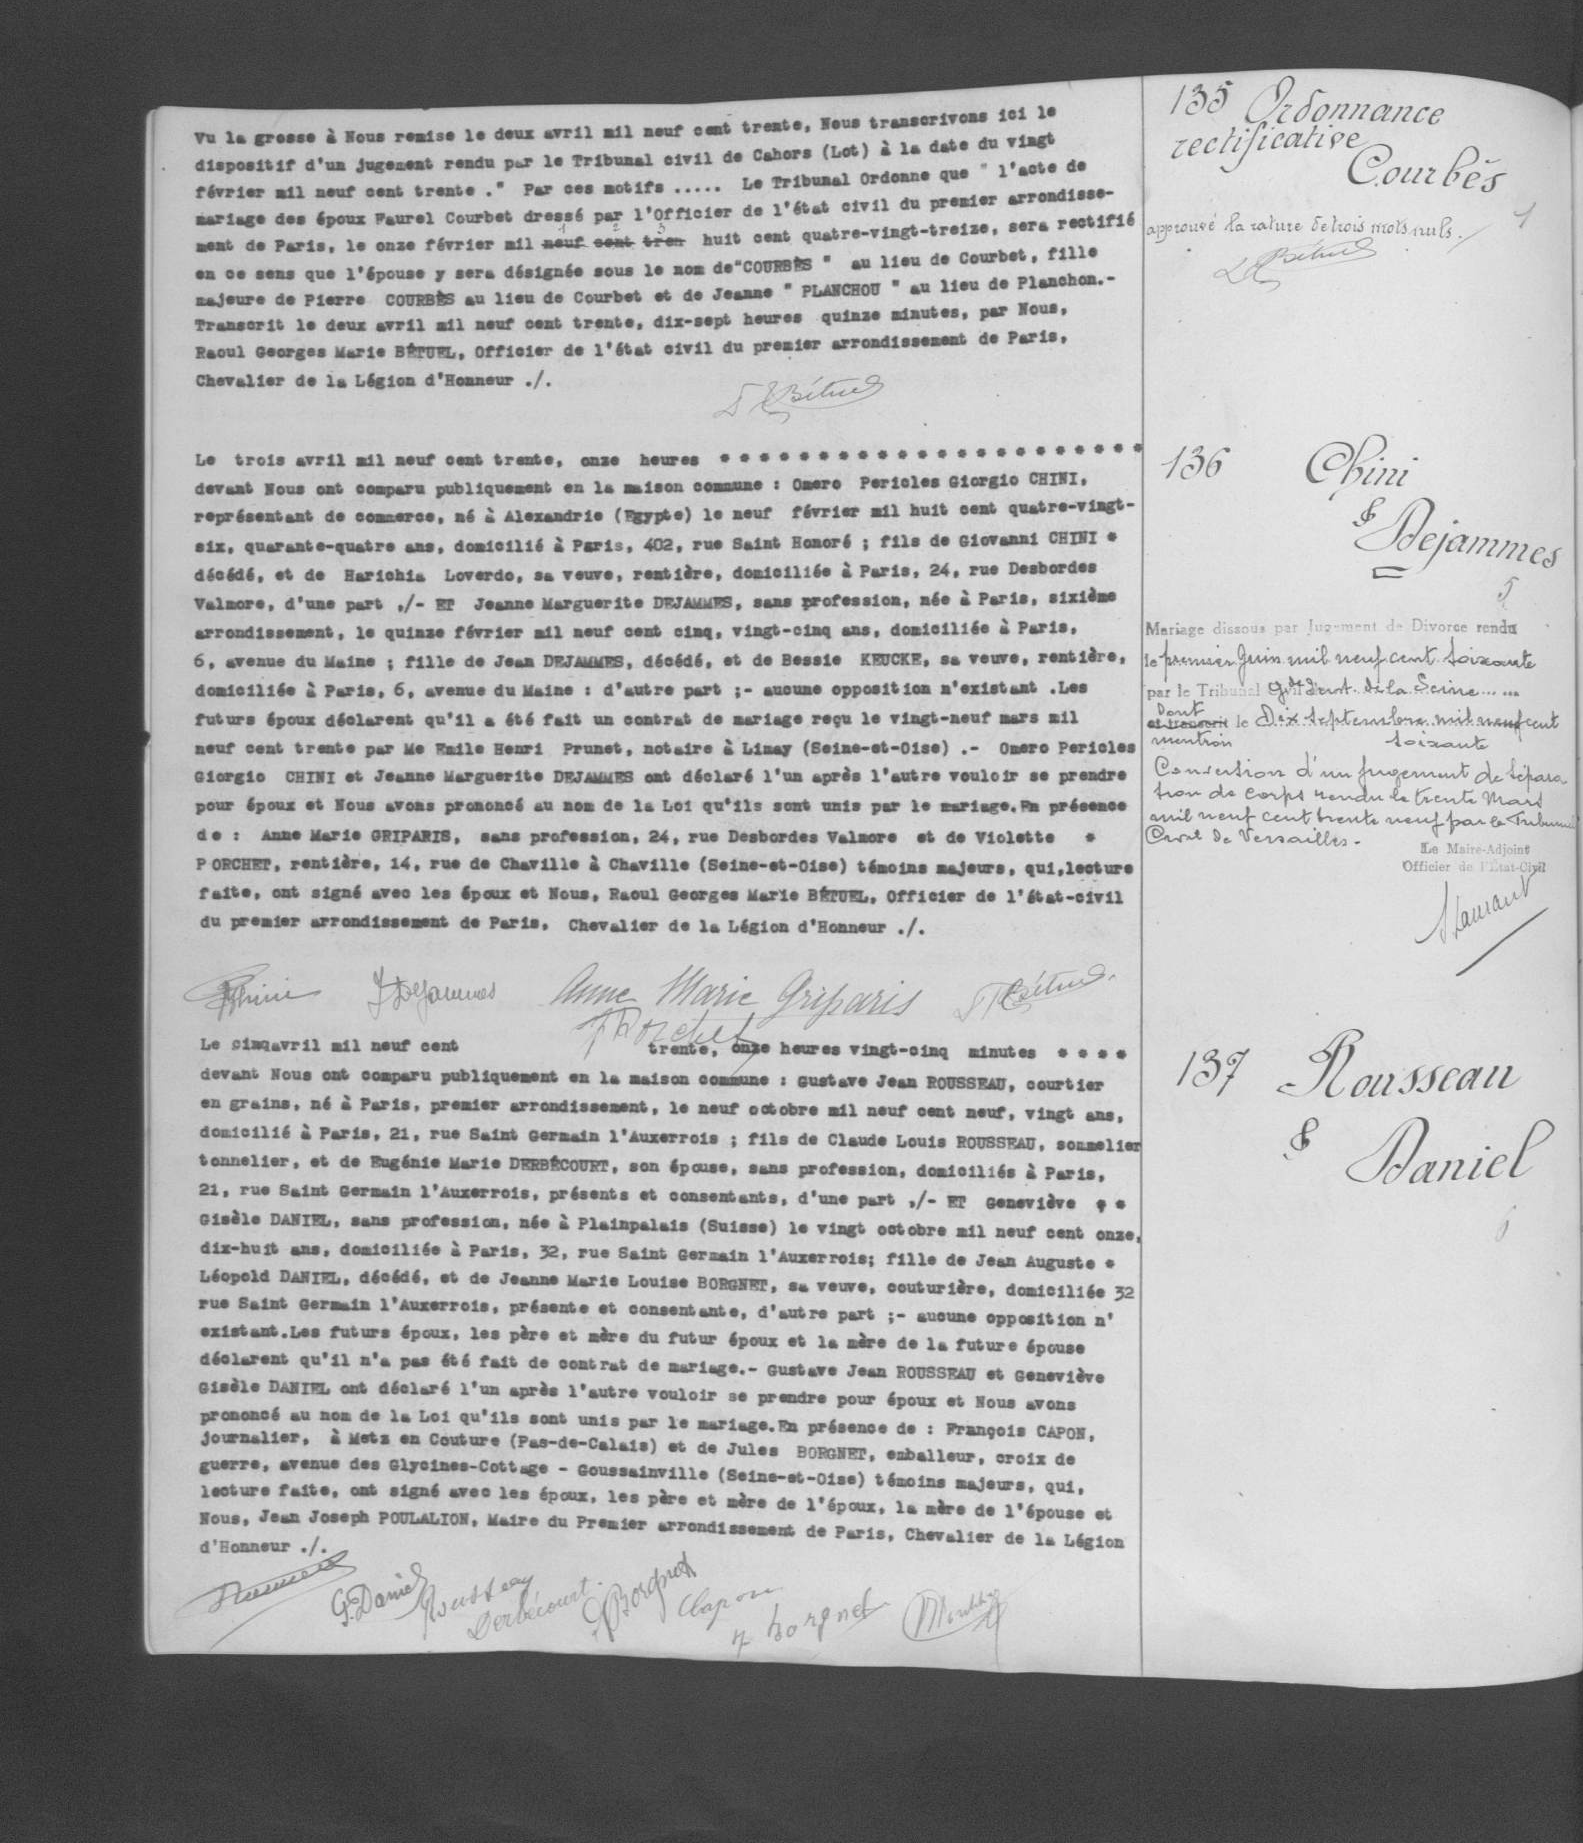Vu la grosse à Nous remise le deux avril mil neuf cent trente, Nous transcrivons ici le
dispositif d'un jugement rendu par le Tribunal civil de Cahor (Lot) à la date du vingt
février mil neuf cent trente." Par ces motifs..... Le Tribunal Ordonne que l'acte de
mariage des époux Faurel Courbet dressé par l'Officier de l'état civil du premier arrondisse-
ment de Paris, le onze février mil neuf cent tren huit cent quatre-vingt-treize, sera rectifié
en ce sens que l'épouse y sera désignée sous le nom de" COURBES" au lieu de Courbet, fille
majeure de Pierre COURBES au lieu de Courbet et de Jeanne" PLANCHOU" au lieu de Planchon .-
Transcrit le deux avril mil neuf cent trente, dix-sept heures quinze minutes, par Nous,
Raoul Georges Marie BETUEL, Officier de l'état civil du premier arrondissement de Paris,
Chevalier de la Légion d'Honneur ./.
Le trois avril mil neuf cent trente, onze heures ****
devant Nous ont comparu publiquement en la maison commune: Omero Pericles Giorgio CHINI,
représentant de commerce, né à Alexandrie (Egypte) le neuf février mil huit cent quatre vingt
six, quarante-quatre ans, domicilié à Paris, 402, rue Saint Honoré; fils de Giovanni CHINI *
décédé, et de Harichia Loverdo, sa veuve, rentière, domiciliée à Paris, 24, rue Desbordes
Valmore, d'une part ,/-ET Jeanne Marguerite DEJAMMES, sans profession, née à Paris, sixième
arrondissement, le quinze février mil neuf cent cinq, vingt-cinq ans, domiciliée à Paris,
6, avenue du Maine; fille de Jean DEJAMMES, décédé, et de Besssie KEUCKE, sa veuve, rentière,
domiciliée à Paris, 6, avenue du Maine: d'autre part ;-aucune opposition n'existant .Les
futurs époux déclarent qu'il a été fait un contrat de mariage reçu le vingt-neuf mars mil
neuf cent trente par Me Emile Henri Prunet, notaire à Limay (Seine-et-Oise) .-Omero Pericles
Giorgio CHINI et Jeanne Marguerite DEJAMMES ont déclaré l'un après l'autre vouloir se prendre
pour époux et Nous avons prononcé au nom de la Loi qu'il sont unis par le mariage. En présence
de: Anne Marie GRIPARIS, sans profession, 24, rue Desbordes Valmore et de Violette *
PORCHET, rentière, 14, rue de Chaville à Chaville (Seine-et-Oise) témoins majeurs, qui, lecture
faite, ont signé avec les époux et Nous, Raoul Georges Marie BETUEL, Officier de l'état-civil
du premier arrondissement de Paris, Chevalier de la Légion d'Honneur ./.
Le cinq avril mil neuf cent trente, onze heures vingt-cinq minutes ****
devant Nous ont comparu publiquement en la maison commune: Gustave Jean ROUSSEAU, courtier
en grains, né à Paris, premier arrondissement, le neuf octobre mil neuf cent neuf, vingt ans,
domicilié à Paris, 21, rue Saint Germain l'Auxerrois; fils de Claude Louis ROUSSEAU, sommelier
tonnelier, et de Eugénie Marie DERBECOURT, son épouse, sans profession, domiciliés à Paris,
21, rue Saint Germain l'Auxerrois, présents et consentants, d'une part ,/-ET Geneviève * *
Gisèle DANIEL, sans profession, née à Plainpalais (Suisse) le vingt octobre mil neuf cent onze,
dix-huit ans, domiciliée à Paris, 32, rue Saint Germain l'Auxerrois; fille de Jean Auguste *
Léopold DANIEL, décédé, et de Jeanne Marie Louise BORGNET, sa veuve, couturière, domiciliée
rue Saint Germain l'Auxerrois, présente et consentante, d'autre part ;-aucune opposition n'
existant. Les futurs époux, les père et mère du futur époux et la mère de la future épouse
déclarent qu'il n'a pas été fait de contat de mariage .-Gustave Jean ROUSSEAU et Geneviève
Gisèle DANIEL ont déclaré l'un après l'autre vouloir se prendre pour époux et Nous avons
prononcé au nom de la Loi qu'ils sont unis par le mariage. En présence de: François CAPON,
journalier, à Metz en Couture (Pas-de-Calais) et de Jules BORGNET, emballeur, croix de
guerre, avenue des Glycines-Cottage-Goussainville (Seine-et-oise) témoins majeurs, qui,
lecture faite, ont signé avec les époux, les père et mère de l'époux, la mère de l'épouse et
Nous, Jean Joseph POULALION, Maire du Premier arrondissement de Paris, Chevalier de la Légion
d'Honneur ./.
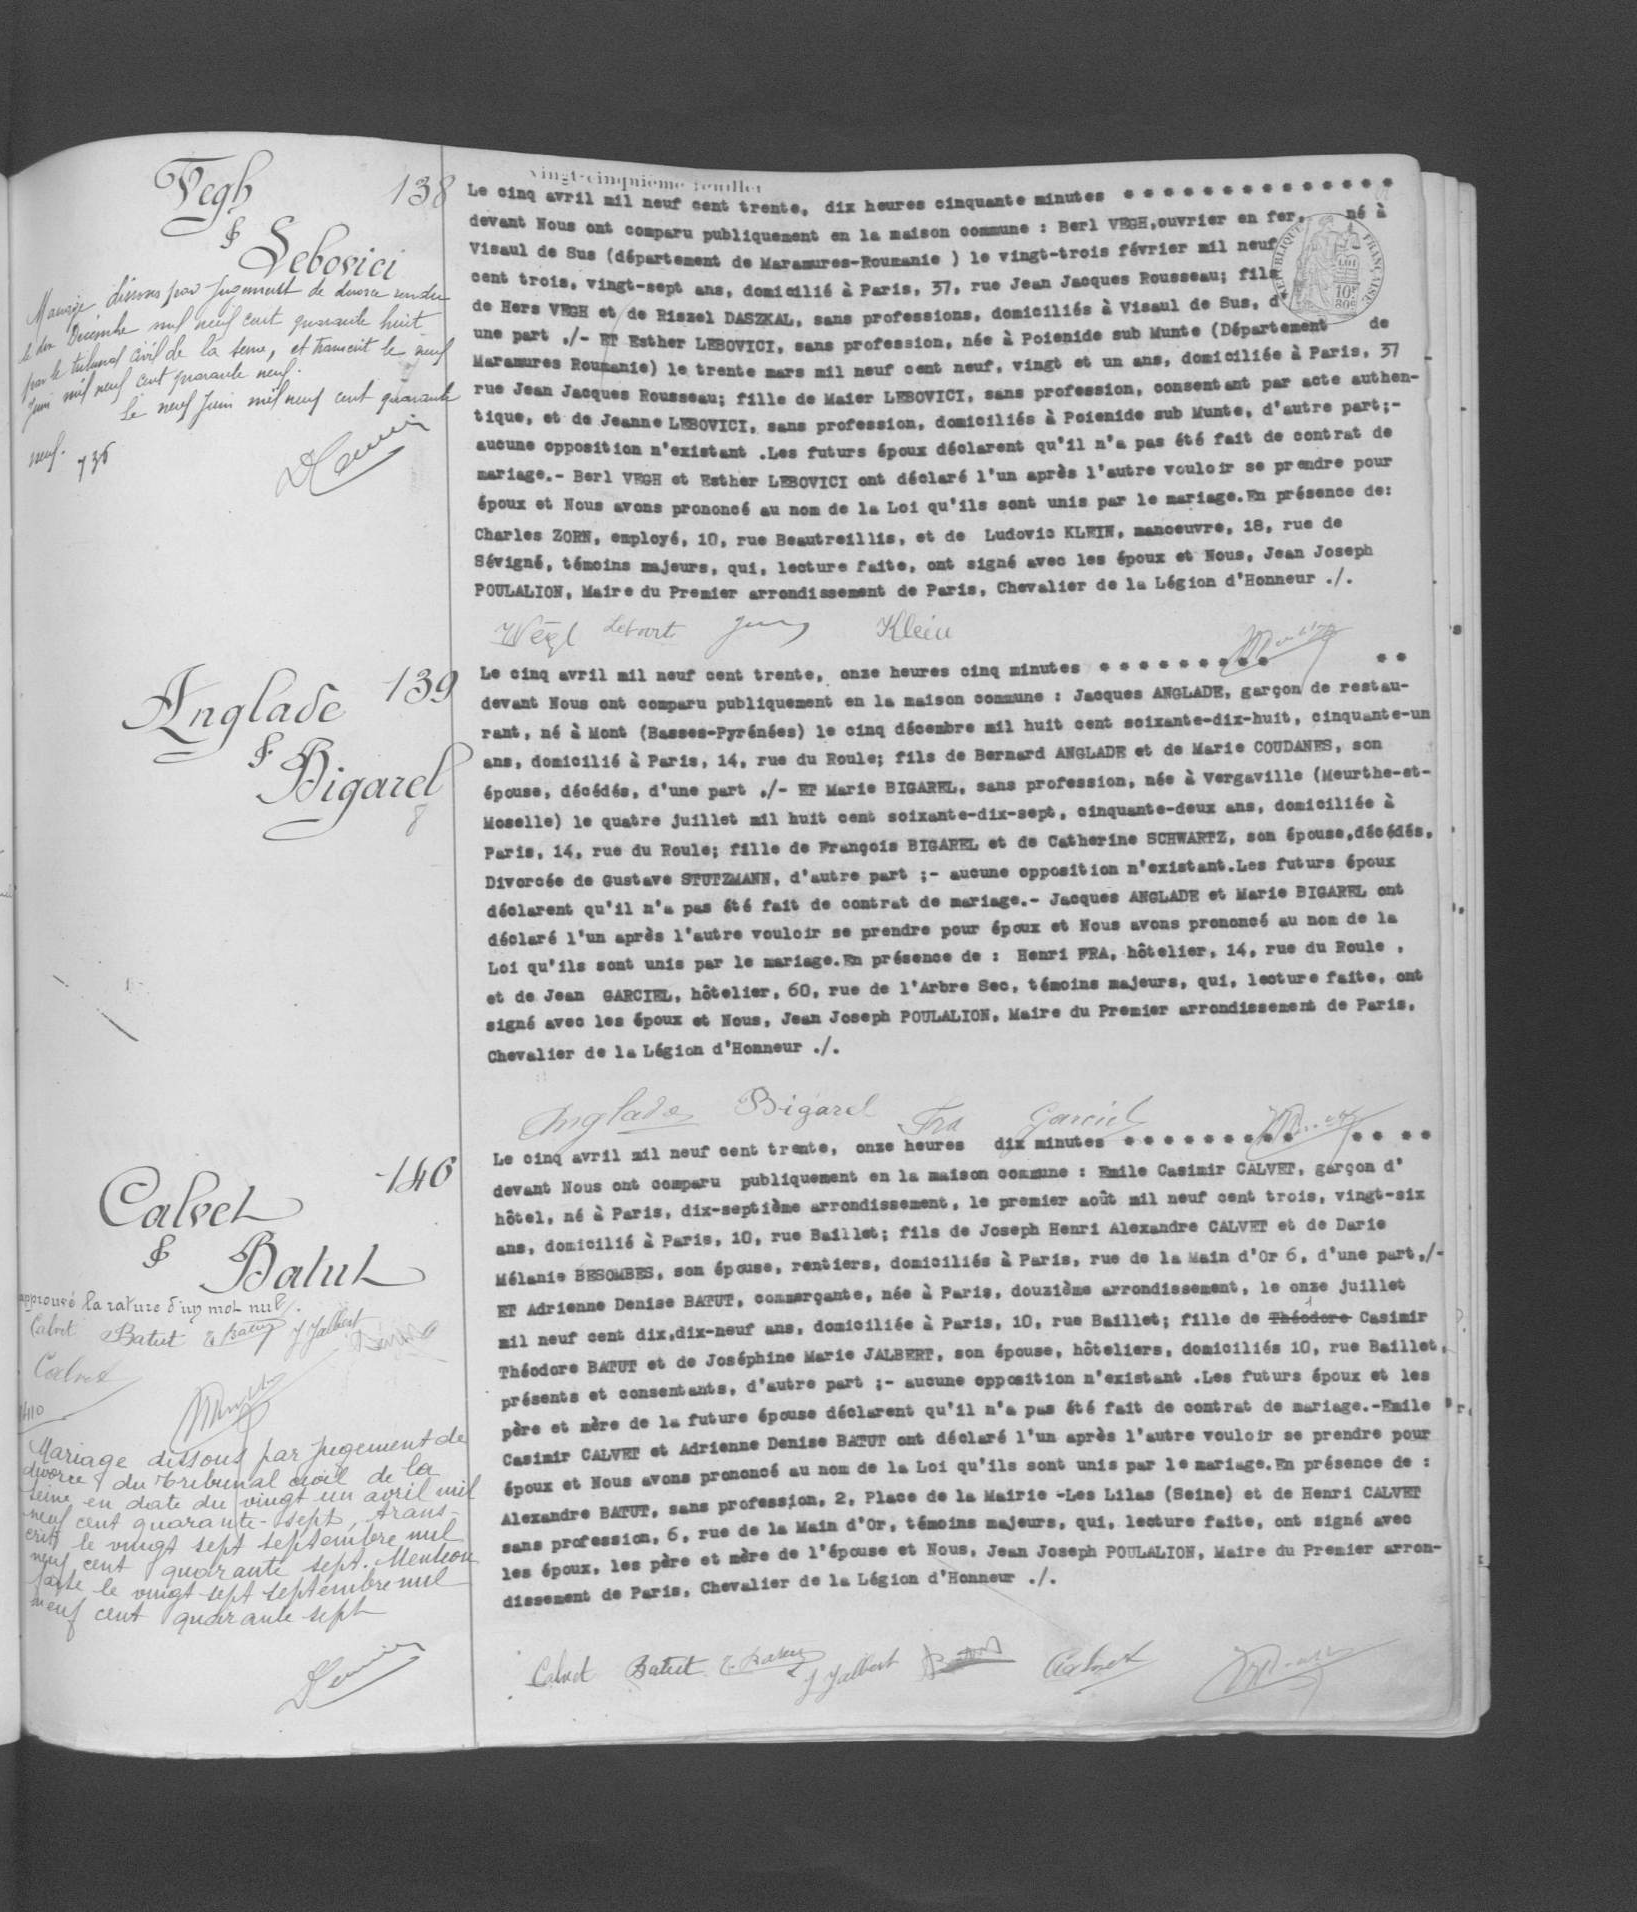Le cinq avril mil neuf cent trente, dix heures cinquante minutes ****
devant Nous ont comparu publiquement en la maison commune: Berl VEGH, ouvrier en fer, né à
Visaul de Sus (département de Maramures-Roumanie) le vingt-trois février mil neuf
cent trois, vingt-sept ans, domicilié à Paris, 37, rue Jean Jacques Rousseau; fils
de Hers VEGH et de Riszel DASZKAL, sans professions, domiciliés à Visaul de Sus, d'
une part ,/-ET Esther LEBOVICI, sans profession, née à Poienide sub Munte (Département de
Maramues Roumanie) le trente mars mil neuf cent neuf, vingt et un ans, domiciliée à Paris, 37
rue Jean Jacques Rousseau; fille de Maier LEBOVICI, sans profession, consentant par acte authen-
tique, et de Jeanne LEBOVICI, sans profession, domiciliés à Poienide sub Munte, d'autre part ;-
aucune opposition n'existant. Les futurs époux déclarent qu'il n'a pas été fait de contrat de
mariage .-Berl VEGH et Esther LEBOVICI ont déclaré l'un après l'autre vouloir se prendre pour
époux et Nous avons prononcé au nom de la Loi qu'ils sont unis par le mariage. En présence de:
Charles ZORN, employé, 10, rue Beautreillis, et de Ludovic KLEIN, manoeuvre, 18, rue de
Sévigné, témoins majeurs, qui, lecture faite, ont signé avec les époux et Nous, Jean Joseph
POULALION, Maire du Premier arrondissement de Paris, Chevalier de la Légion d'Honneur ./.
Le cinq avril mil neuf cent trente, onze heures cinq minutes ****
devant Nous ont comparu publiquement en la maison commune: Jacques ANGLADE, garçon de restau-
rant, né à Mont (Basses-Pyrénées) le cinq décembre mil huit cent soixante-dix-huit, cinquante-un
ans, domicilié à Paris, 14, rue du Roule; fils de Bernard ANGLADE et de Marie COUDANES, son
épouse, décédés, d'une part ,/-ET Marie BIGAREL, sans profession, née à Vergaville (Meurthe-et-
Moselle) le quatre juillet mil huit cent soixante-dix-sept, cinquante-deux ans, domiciliée à
Paris, 14, rue du Roule; fille de François BIGAREL et de Catherine SCHWARTZ, son épouse, décédés,
Divorcée de Gustave STUTZMANN, d'autre part ;-aucune opposition n'existant. Les futurs époux
déclarent qu'il n'a pas été fait de contrat de mariage .-Jacques ANGLADE et Marie BIGAREL ont
déclaré l'un après l'autre vouloir se prendre pour époux et Nous avons prononcé au nom de la
Loi qu'ils sont unis par le mariage. En présence de: Henri FRA, hôtelier, 14, rue du Roule,
et de Jean GARCIEL, hôtelier, 60, rue de l'Arbre Sec, témoins majeurs, qui, lecture faite, ont
signé avec les époux et Nous, Jean Joseph POULALION, Maire du Premier arrondissement de Paris,
Chevalier de la Légion d'Honneur ./.
Le cinq avril mil neuf cent trente, onze heures dix minutes ****
devant Nous ont comparu publiquement en la maison commune: Emile Casimir CALVET, garçon d'
hôtel, né à Paris, dix-septième arrondissement, le premier août mil neuf cent trois, vingt-six
ans, domicilié à Paris, 10, rue Baillet; fils de Joseph Henri Alexandre CALVET et de Darie
Mélanie BESOMBES, son épouse, rentiers, domiciliés à Paris, rue de la Main d'Or 6, d'une part ,/-
ET Adrienne Denise BATUT, commerçante, née à Paris, douzième arrondissement, le onze juillet
mil neuf cent dix, dix-neuf ans, domiciliée à Paris, 10, rue Baillet; fille de Théodore Casimir
Théodore BATUT et de Joséphine Marie JALBERT, son épouse, hôteliers, domiciliés 10, rue Baillet,
présents et consentants, d'autre part ;-aucune opposition n'existant. Les futurs époux et les
père et mère de la future épouse déclarent qu'il n'a pas été fait de contrat de mariage .-Emile
Casimir CALVET et Adrienne Denise BATUT ont déclaré l'un après l'autre vouloir se prendre pour
époux et Nous avons prononcé au nom de la Loi qu'ils sont unis par le mariage. En présence de:
Alexandre BATUT, sans profession, 2, Place de la Mairie-Les Lilas (Seine) et de Henri CALVET
sans profession, 6, rue de la Main d'Or, témoins majeurs, qui, lecture faite, ont signé avec
les époux, les père et mère de l'épouse et Nous, Jean Joseph POULALION, Maire du Premier arron-
dissement de Paris, Chevalier de la Légion d'Honneur ./.